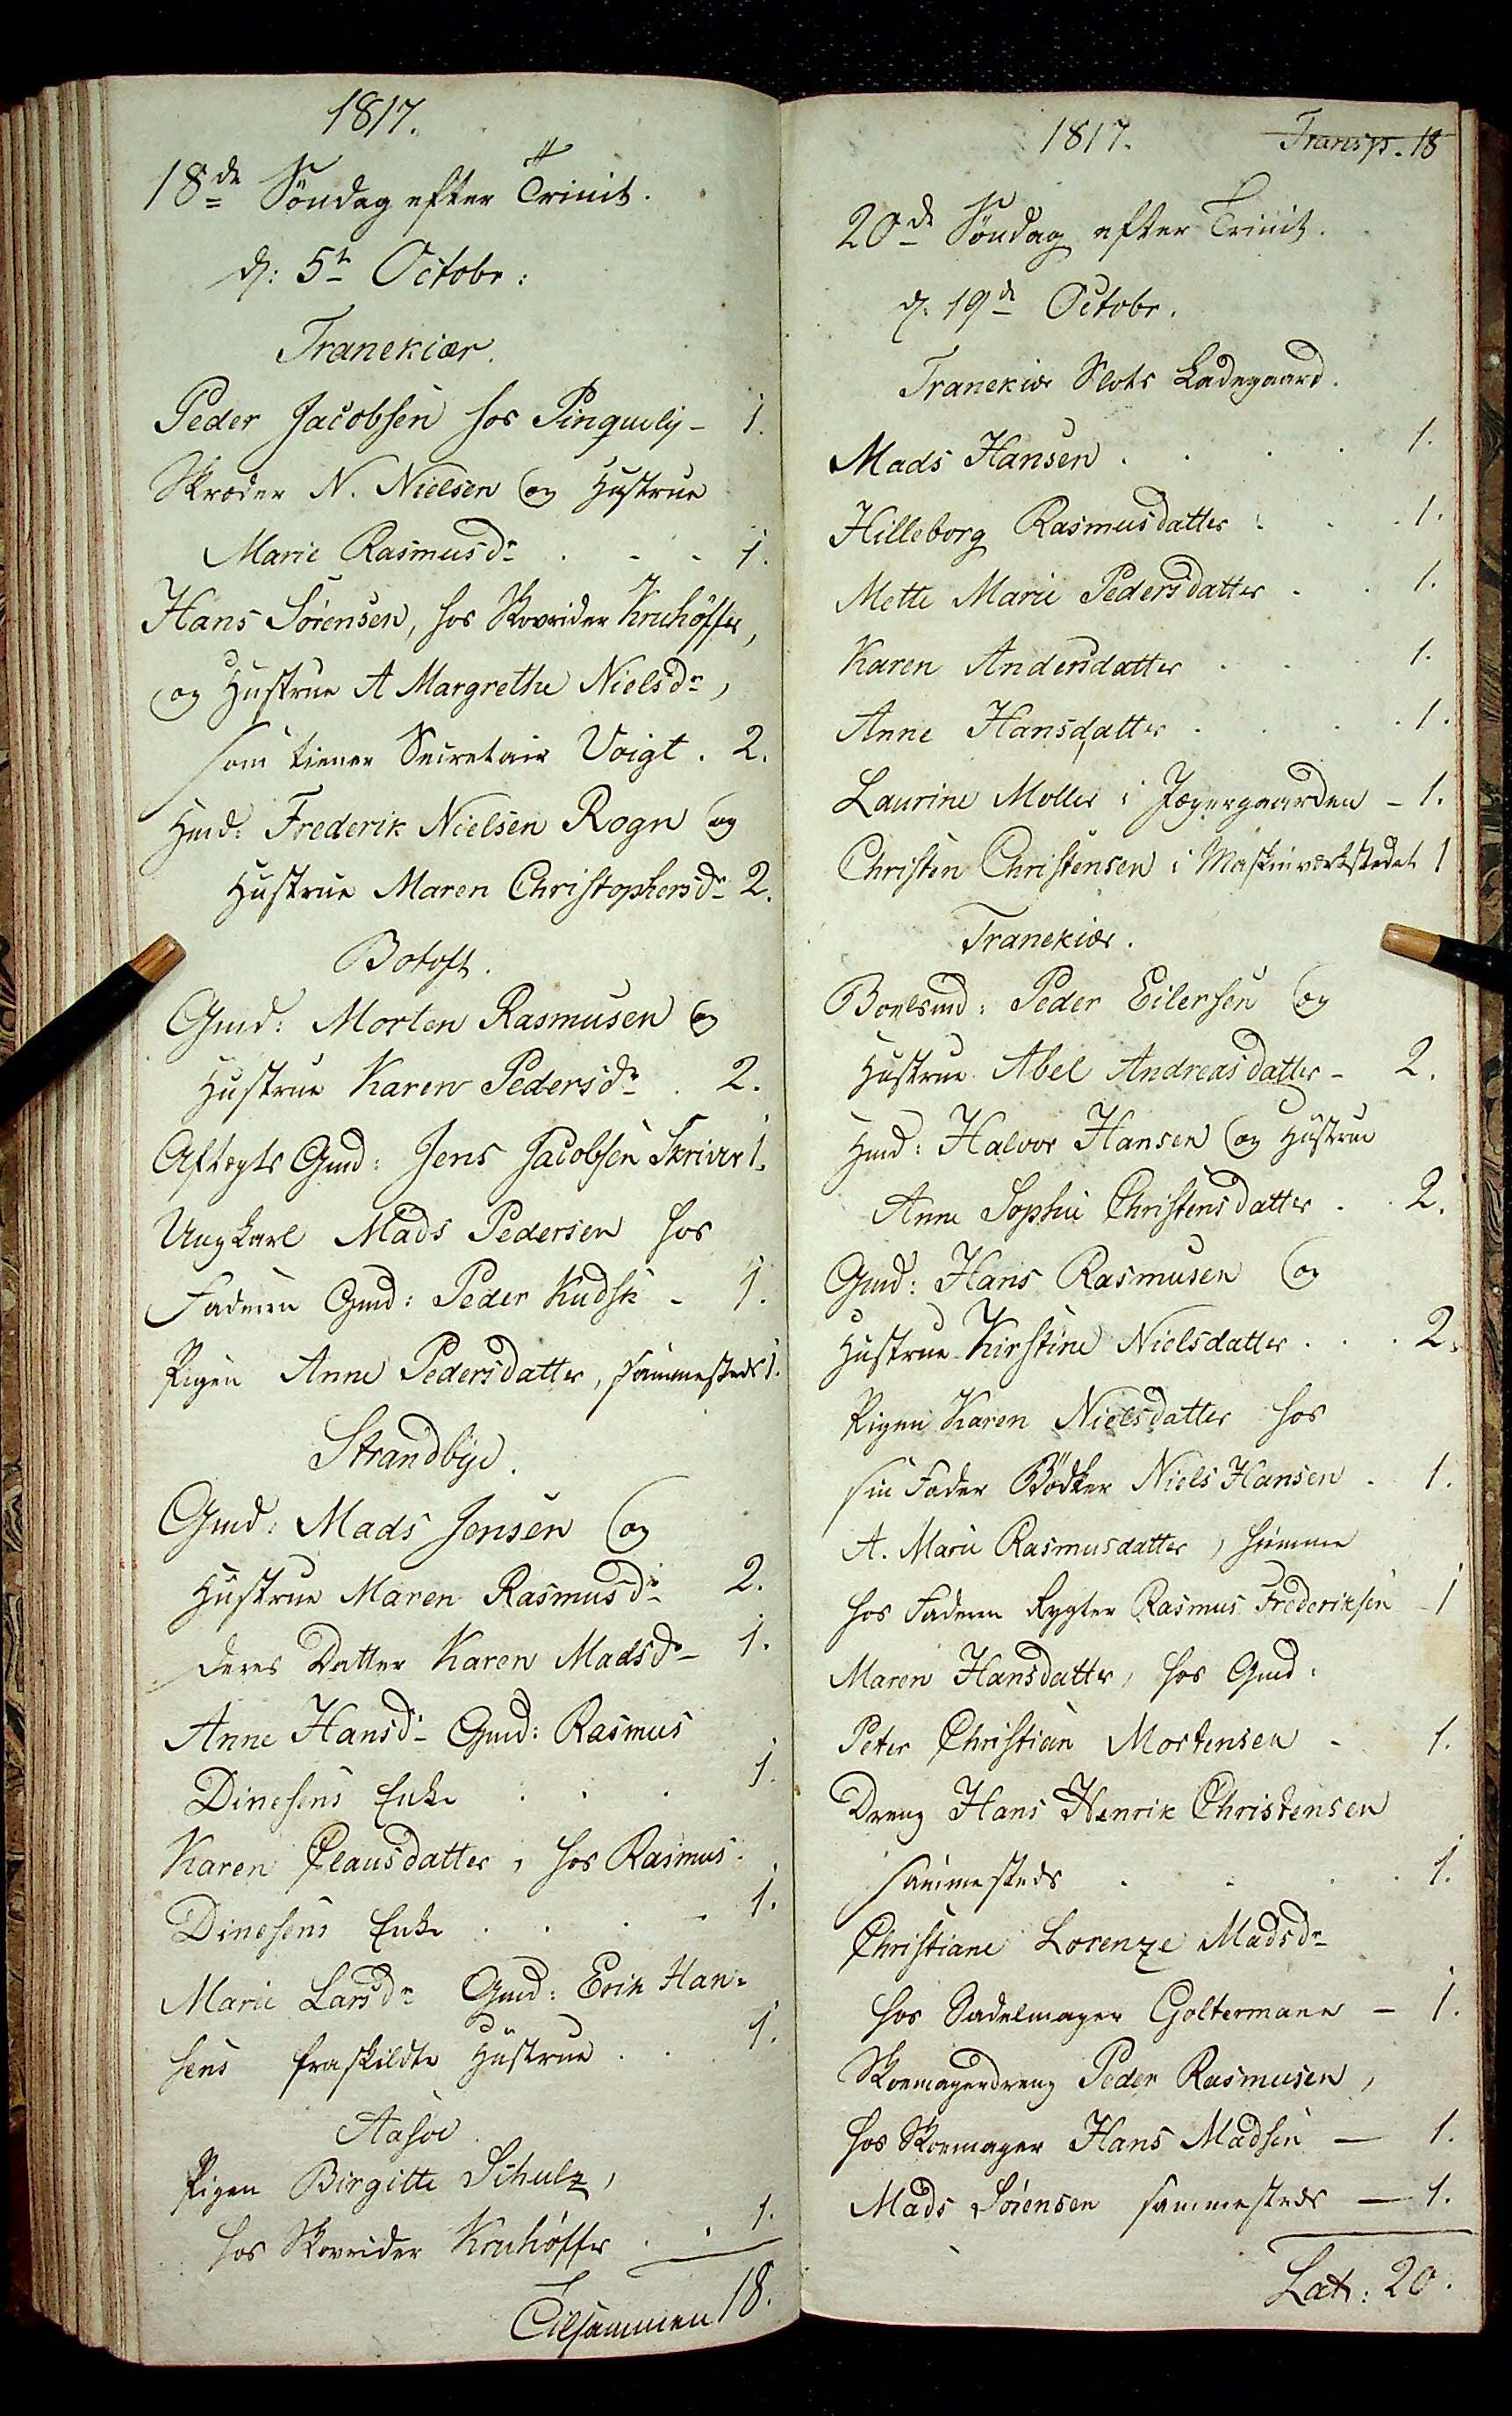1817.
18de Søndag efter Trinit.
d: 5te Octobr:
Tranekiær.
Peder Jacobsen hos Pinquely
Skræder N. Nielsen og Hustrue
Marie Rasmusdr . . . 1.
Hans Sørensen, hos Skovrider Kruhøffer
og Hustrue A. Margrethe Nielsdr
som tiener Secretair Voigt . 2.
Hmd: Frederik Nielsen Rogn og
Hustrue Maren Christophersdr 2.
Botoft
Gmd: Morten Rasmusen og
Hustrue Karen Pedersdr  . 2.
Aftægts Gmd: Jens Jacobsen Skriver 1.
Ungkarl Mads Pedersen hos
Faderen Gmd: Peder Kudsk - 1.
Pigen Anne Pedersdatter, sammested 1
Strandbye.
Gmd: Mads Jensen og
Hustrue Maren Rasmusdr 2.
deres Datter Karen Madsdr 1.
Anne Hansdr Gmd: Rasmus
Dinesens Enke . . . 1.
Karen Clausdatter, hos Rasmus
Dinesens Enke . . . - 1.
Marie Larsdr Gmd: Erik Han¬
sens fraskildte Hustrue . . .1.
Aasøe
Pigen Birgitte Schulz,
hos Skovrider Kruhøffer . . 1.
Tilsammen 18
1817.
Transp . 18
20de Søndag efter Trinit.
d: 19de Octobr.
Tranekiærr Slots Ladegaard.
Mads Hansen . . . . 1.
Hilleborg Rasmusdatter . . . 1.
Mette Marie Pedersdatter . . 1.
Karen Andersdatter . . . 1.
Anne Hansdatter . . . . 1.
Laurine Møller i Jægergaarden - 1.
Christen Christensen i Maskinværkstedet 1
Tranekiær.
Boelsmd: Peder Eilersen og
Hustrue Abel Andreasdatter - 2.
Hmd: Halvor Hansen og Hustrue
Anne Sophie Christensdatter . . 2.
Gmd: Hans Rasmusen og
Hustrue Kirstine Nielsdatter . . . 2
Pigen Karen Nielsdatter hos
sin Fader Bødker Niels Hansen . 1
A. Marie Rasmusdatter, hiemme
hos Faderen Rygter Rasmus Frideriksen 1
Maren Hansdatter, hos Gmd:
Peter Christian Mortensen - 1.
Dreng Hans Henrik Christensen
sammesteds . . . . 1.
Christiane Lorenze Madsdr
hos Sadelmager Goltermann - 1.
Skoemagerdreng Peder Rasmusen,
hos Skoemager Hans Madsen - 1.
Mads Sørensen sammesteds - 1.
Lat: 20
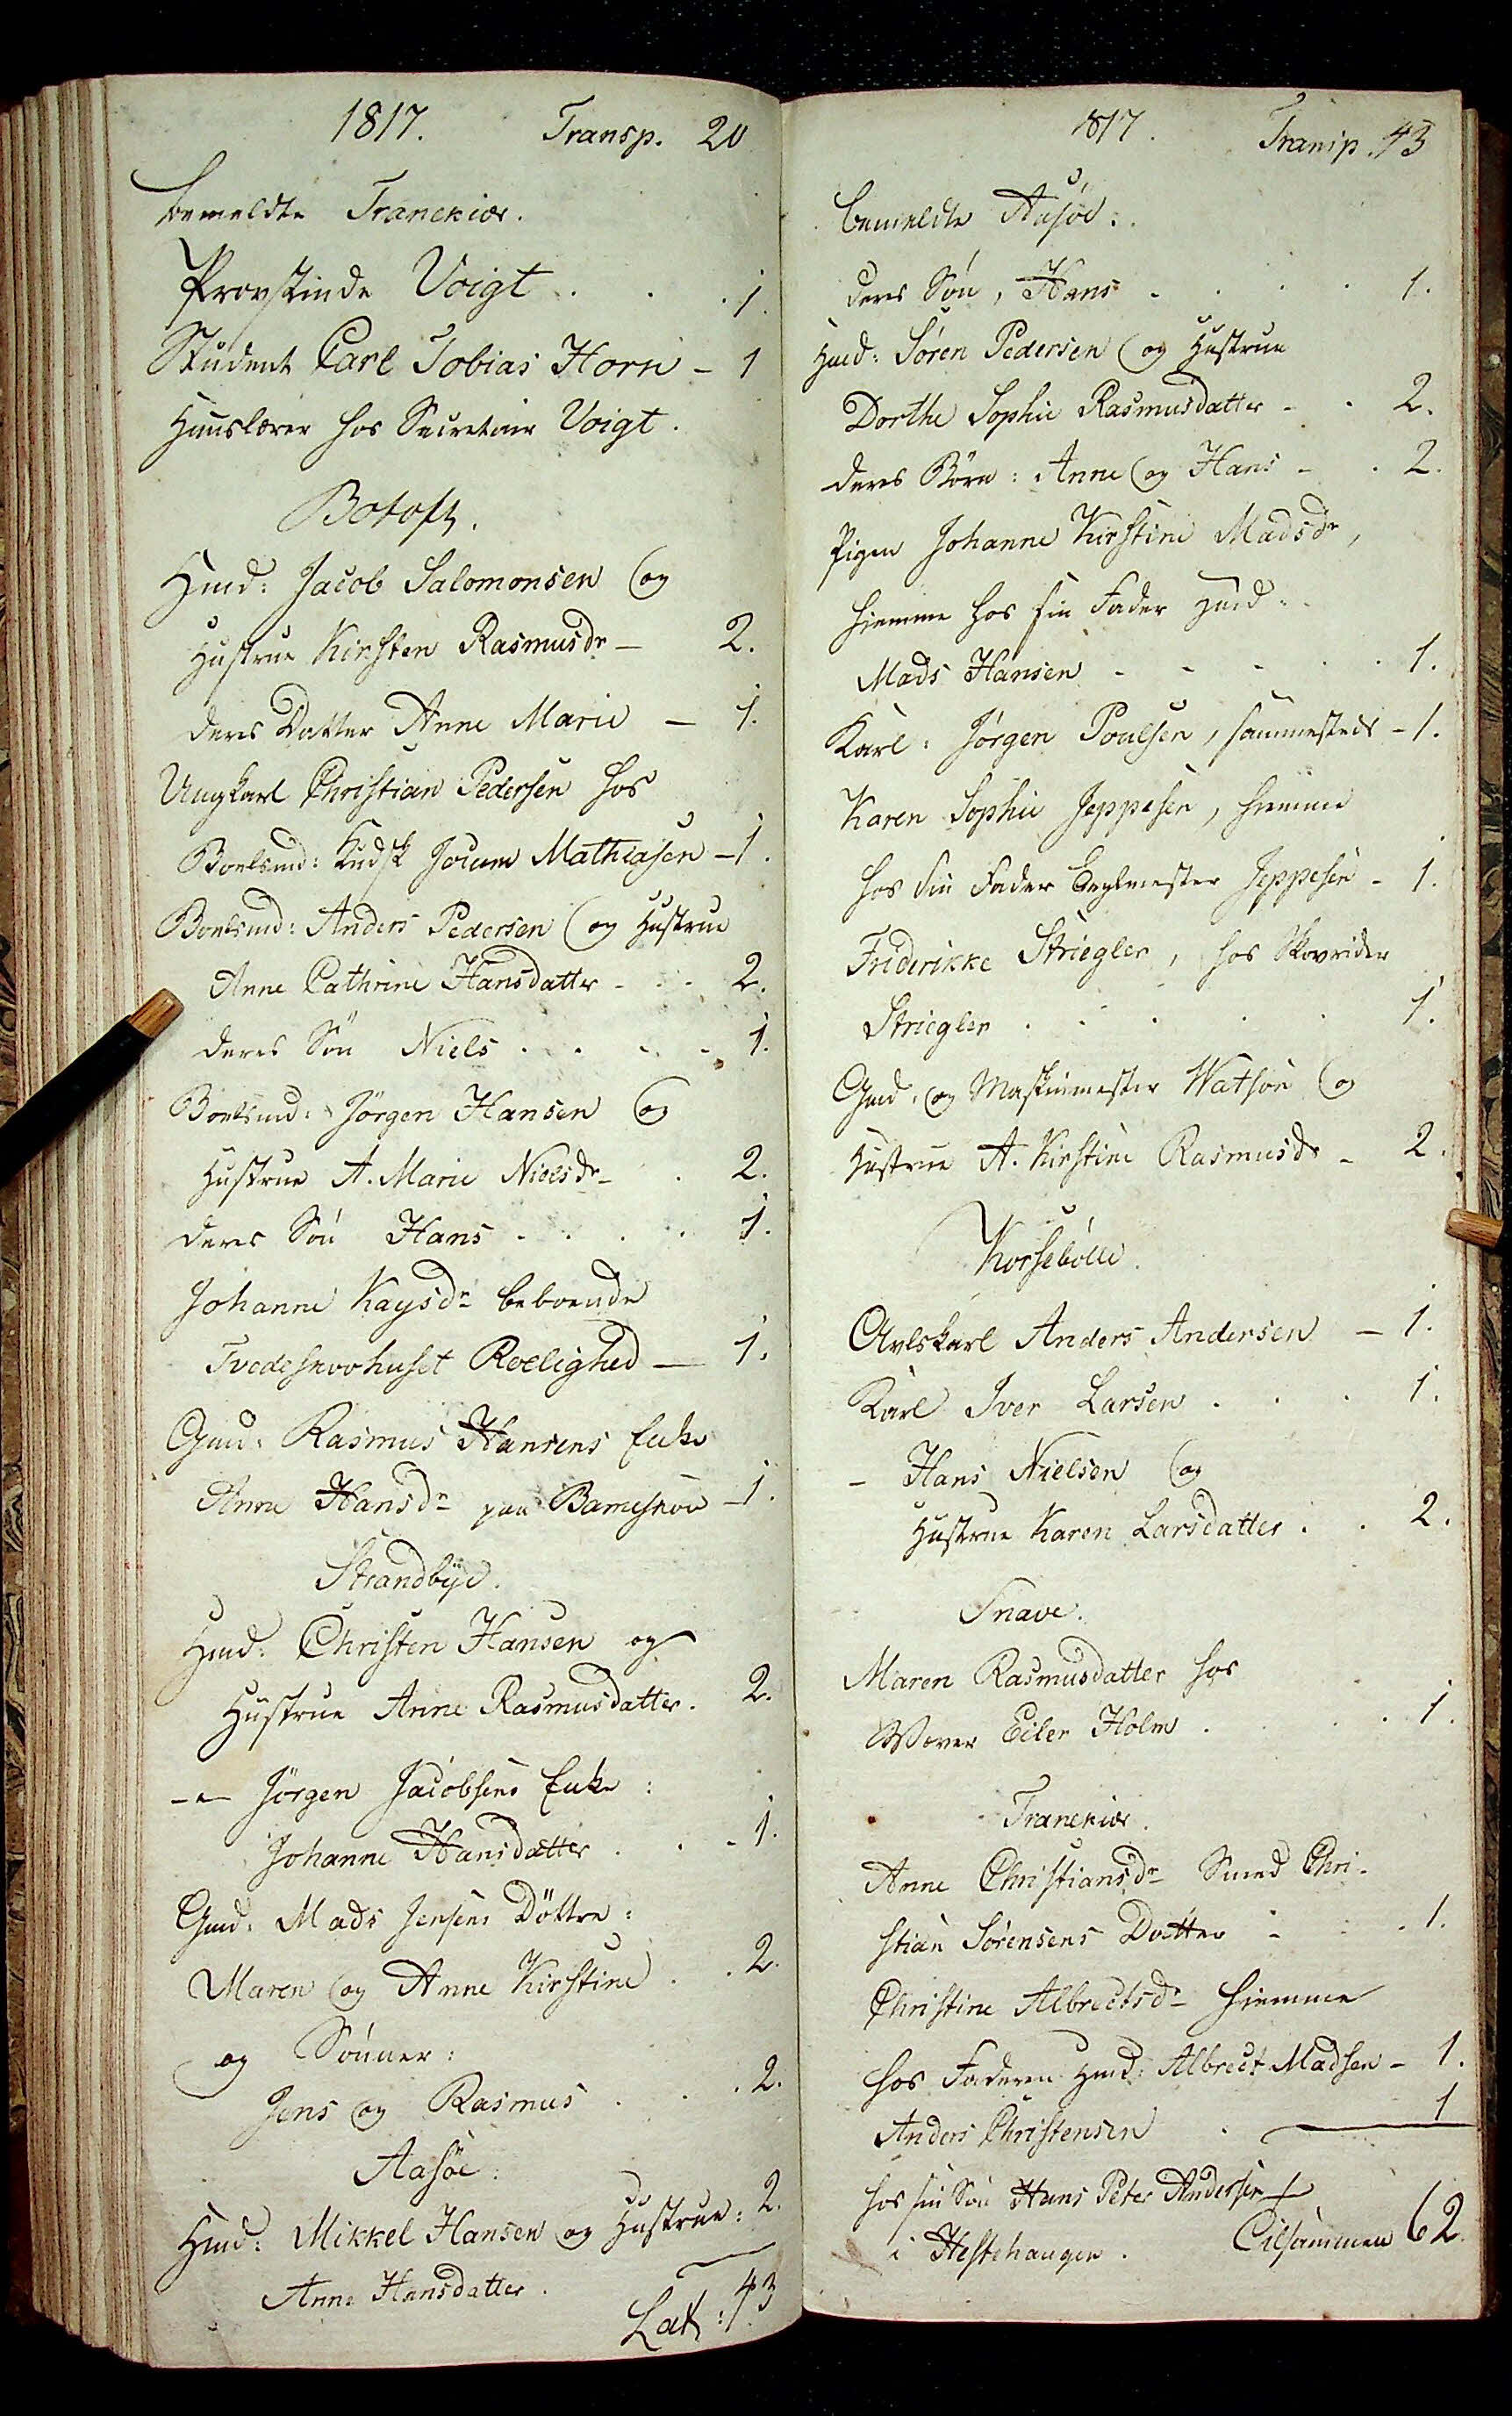1817.
Transp. 20
bemmeldte Tranekiær.
Provstinde Voigt . . . 1
Student Carl Tobias Horn - 1
Huuslærer hos Secretair Voigt.
Botoft.
Hmd: Jacob Salomonsen og
Hustrue Kirsten Rasmusdr - 2.
ders Datter Anne Marie - 1.
Ungkarl Christian Pedersen hos
Boelsd: Kudsk Moder Mathiasen
Boelsmd: Anders Pedersen og Hustrue
Anne Cathrine Hansdatter . . . 2.
deres Søn Niels . . . . 1.
Boelsmd: Jørgen Hansen og
Hustrue A. Marie Nielsdr . 2.
deres Søn Hans . . . . 1
Johanne Kaysdr beboende
Tvedeskovhuset Roelighed - 1.
Gmd: Rasmus Hansens Enke
Anna Hansdr paa Bameskov - 1.
Strandbye.
Hmd: Christen Hansen og
Hustrue Anne Rasmusdatter . 2.
-"- Jørgen Jacobsens Enke
Johanne Hansdatter . . . 1
Gmd: Mads Jensens Døttre:
Maren og Anne Kirstine . . 2.
og Sønner:
Jens og Rasmus . . . 2
Aasøe:
Hmd: Mikkel Hansen og Hustrue : 2
Anne Hansdatter
Lat: 43
1817
Transp. 43
bemeldte Aasøe.
deres Søn, Hans . . . . 1.
Hmd: Søren Pedersen og Hustrue
Dorthe Sophie Rasmusdatter . .  2.
deres Børn: Anne og Hans - . 2.
Pigen Johanne Kirstine Madsdr
hiemme hos sin Fader Hmd:
Mads Hansen . . . . . 1.
Karl: Jørgen Poulsen, sammesteds - 1.
Karen Sophie Jeppesen, hiemme
hos sin Fader Teglmester Jeppesen -1.
Friderikke Striegler, hos Skovrider
Striegler . . . . . 1.
Gmd: og Maskinmester Watson og
Hustrue A. Kirstine Rasmusdr - 2.
Korsebølle
Avlskarl Anders Andersen - 1
Karl Iver Larsen . . . 1.
- Hans Nielsen og
Hustrue Karen Larsdatter . . 2.
Snave.
Maren Rasmusdatter hos
Væver Eiler Holm . . . . 1.
Tranekiær
Anne Christiansdr Smed Chri¬
stian Sørensens Datter : : . 1.
Christine Albrectsdr hiemme
hos Faderen Hmd: Albrect Madsen - 1.
Anders Christensen . 1
hos sin Søn Hans Peter Andersen
i Hestehaugen
Tilsammen 62.